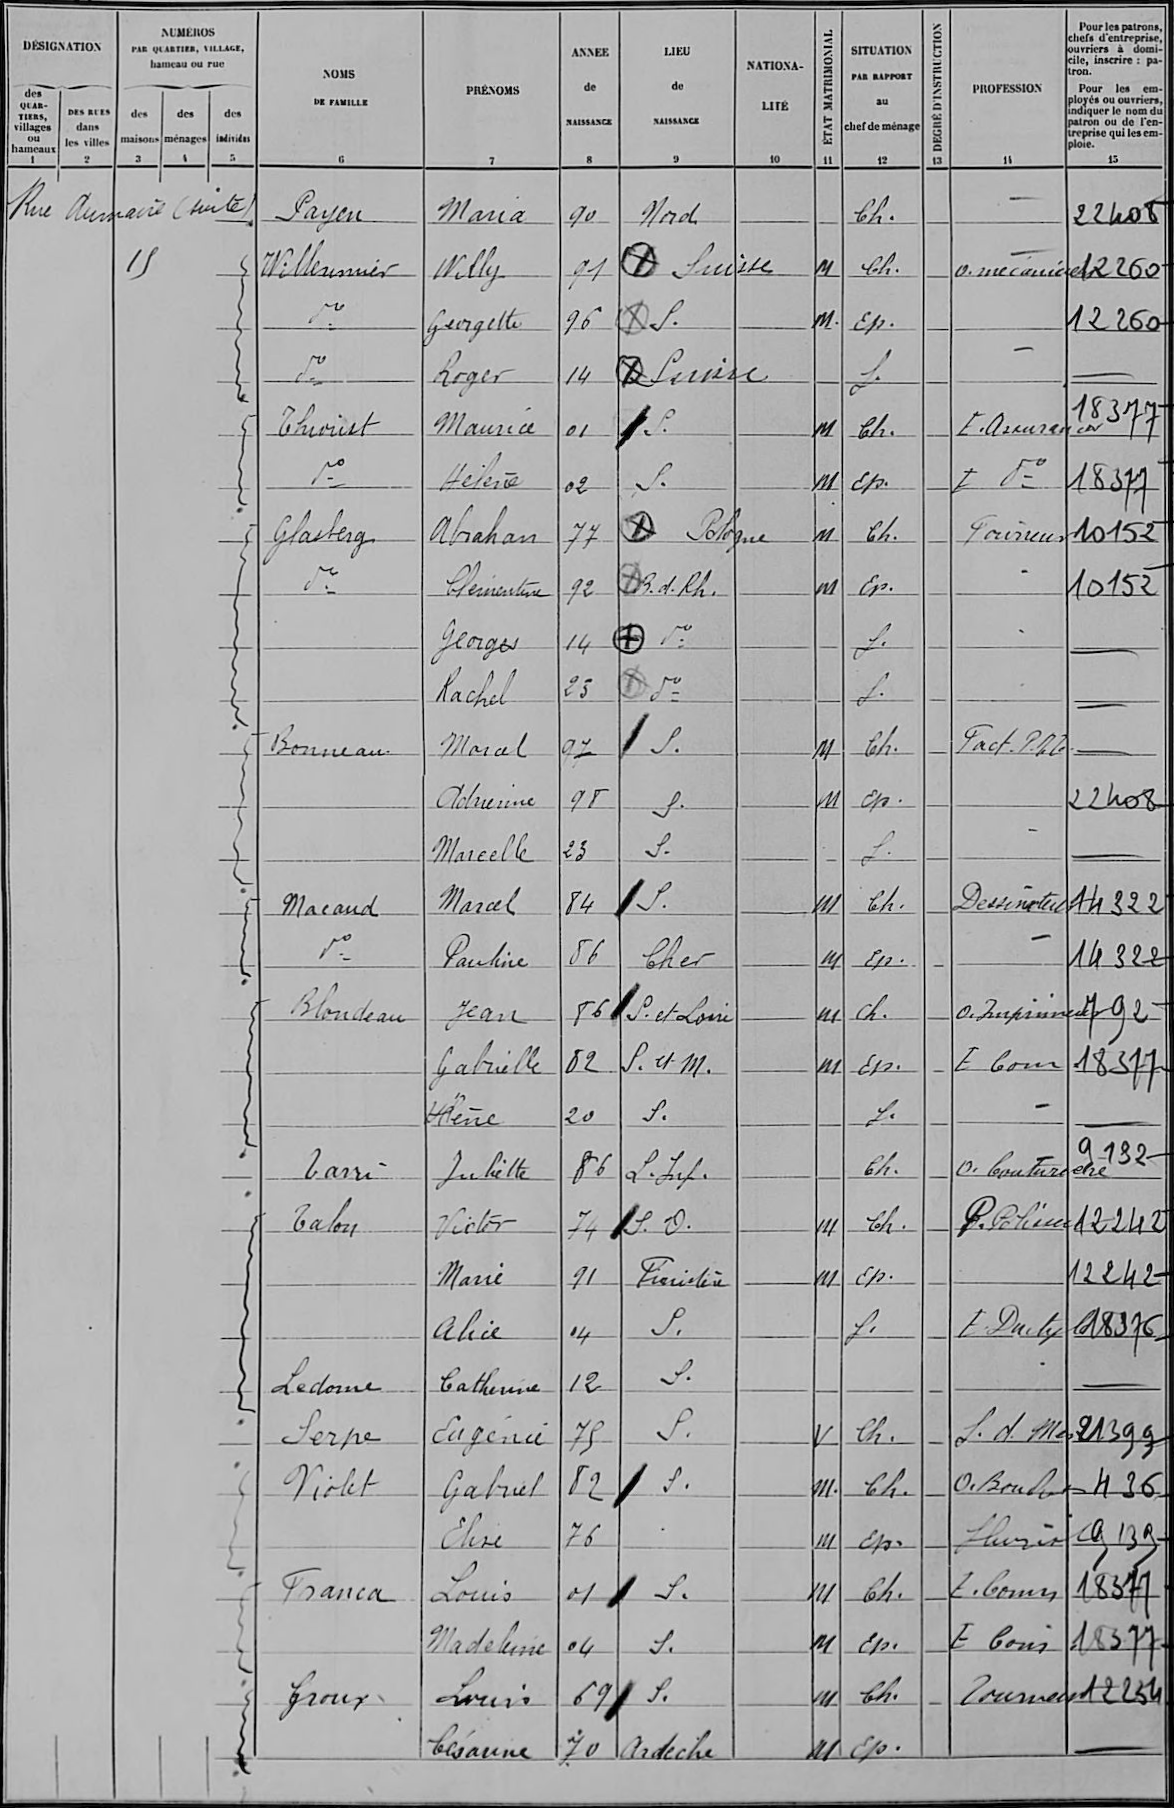Payen/Maria/90/Nord/¤/¤/Ch /¤/¤/22408
Willeumier/Willy/91/Suisse/¤/M/Ch /¤/o mécanicien/12260
d°/Georgette/96/S /¤/M /Ep /¤/¤/12260
d°/Roger/14/Suisse/¤/¤/f /¤/¤/¤
Thivrist/Maurice/01/S /¤/M/Ch /¤/E Assurance/18377
d°/Hélène/02/S /¤/M/Ep /¤/E d°/18377
Glasberg/Abrham/77/Pologne/¤/M/Ch /¤/Fourreur/10152
d°/Clémentine/92/B d Rh /¤/M/ep /¤/¤/10152
¤/Georges/14/d°/¤/¤/f /¤/¤/¤
¤/Rachel/23/d°/¤/¤/S /¤/¤/¤
Bonneau/Marcel/97/S /¤/M/Ch /¤/Fact PTT/¤
¤/Adrienne/98/S /¤/M/ep /¤/¤/22408
¤/Marcelle/23/S /¤/¤/f /¤/¤/¤
Macaud/Marcel/84/S /¤/M/Ch /¤/Dessinateur/14322
d°/Pauline/86/Cher/¤/M/Ep /¤/¤/14322
Blondeau/Jean/86/S et Loire/¤/M/Ch /¤/o Imprimeur/792
¤/Gabrielle/82/S et M /¤/M/Ep /¤/E Com/18377
¤/Hélène/20/S /¤/¤/f /¤/¤/¤
Tarri/Juliette/86/L Inf /¤/¤/Ch /¤/o Couturière/?9132
Talon/Victor/74/S O /¤/M/Ch /¤/P Polisseur/12242
¤/Marie/91/Finistère/¤/M/Ep /¤/¤/12242
¤/Alice/14/S /¤/¤/f /¤/E Dactylo/18376
Ledorne/Catherine/12/S /¤/¤/¤/¤/¤/¤
Serpe/Eugénie/75/S /¤/V/Ch /¤/f d Men/21399
Violet/Gabriel/82/S /¤/M /Ch /¤/O Boucher/436
¤/Elise/76/¤/¤/M/Ep /¤/fleuriste/9139
Franca/Louis/01/S /¤/M/Ch /¤/E Comm/18377
¤/Madeleine/04/S /¤/M/Ep /¤/E Com/18377
Groux/Louis/69/S /¤/M/Ch /¤/tourneur/12254
¤/Césarine/70/Ardèche/¤/M/Ep /¤/¤/¤
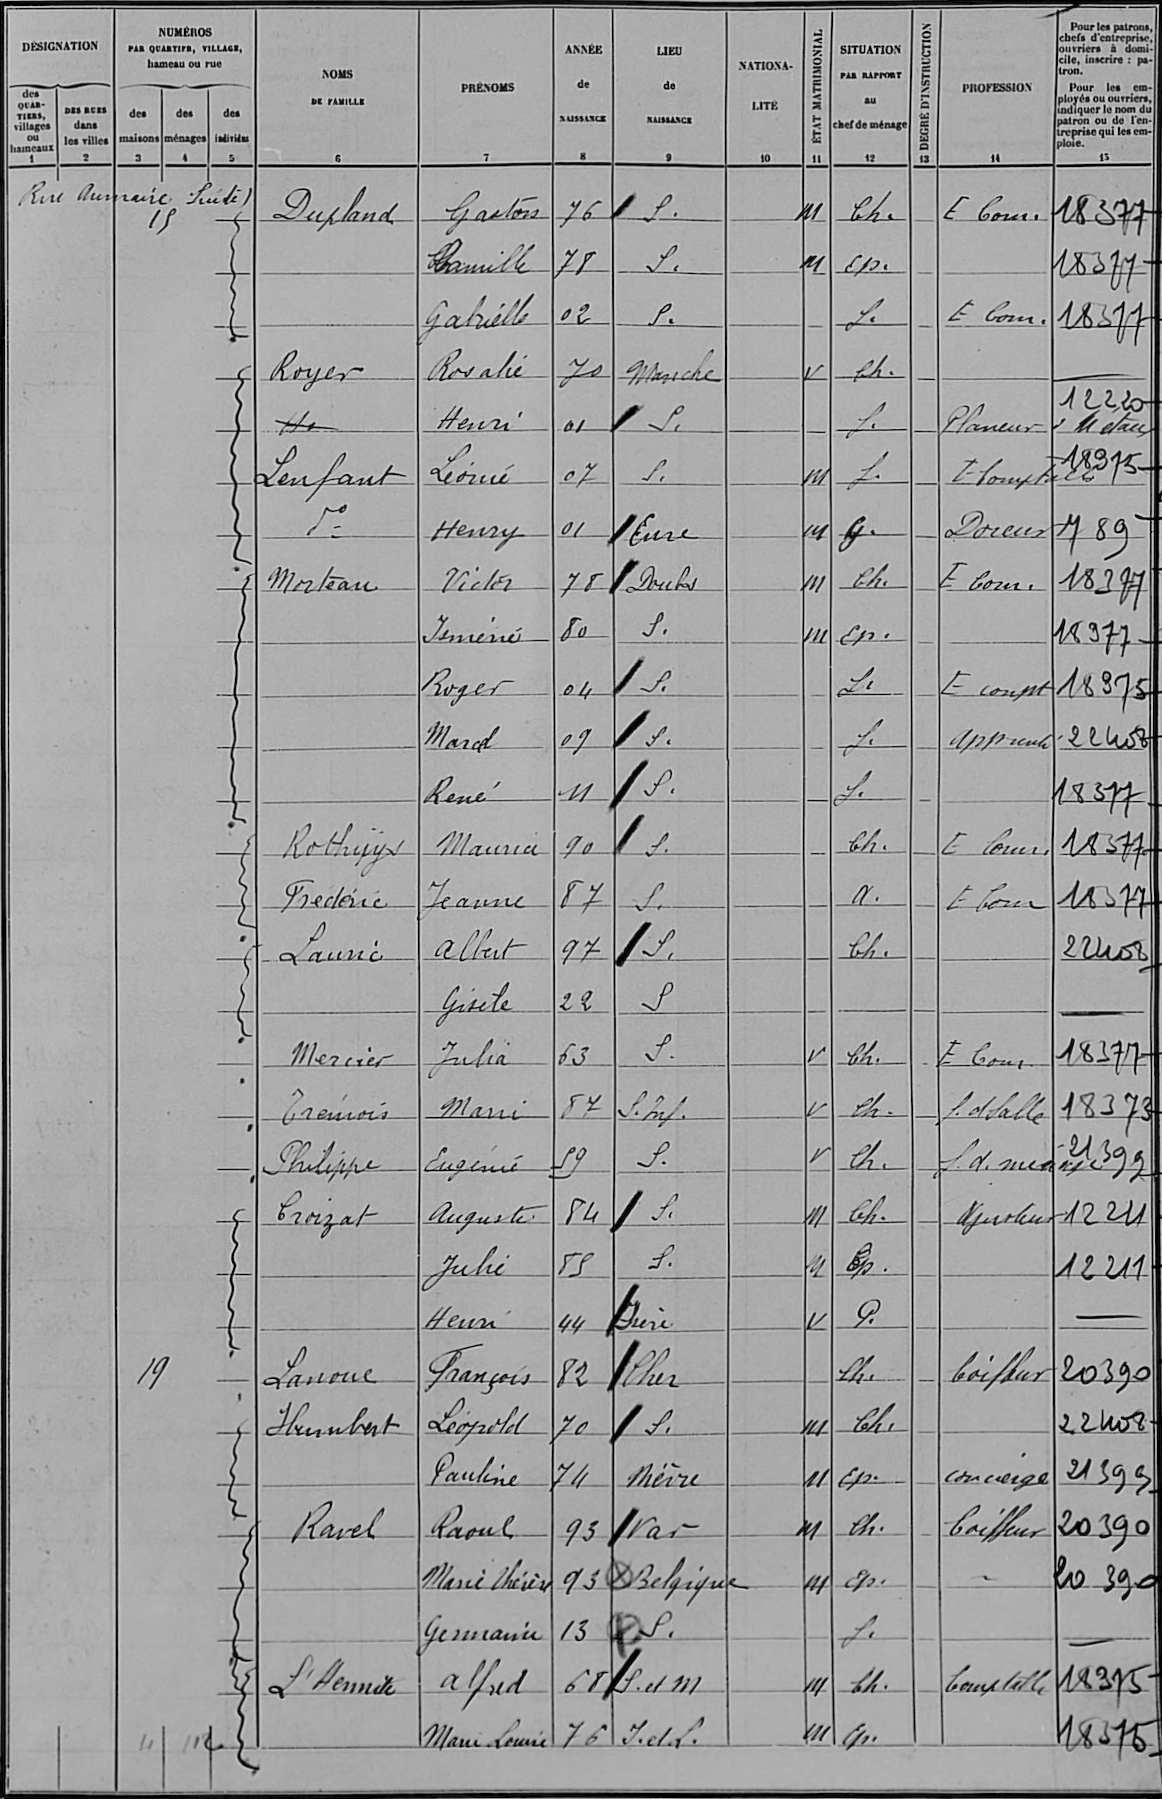Dupland/Gaston/76/S /¤/M/Ch /¤/E Com/18377
¤/Camille/78/S /¤/M/Ep /¤/¤/18377
¤/Gabrielle/02/S /¤/¤/f /¤/E Com /18377
Royer/Rosalie/70/Manche/¤/V /Ch /¤/¤/¤
¤/Henri/01/S /¤/¤/f /¤/Planeur de Métaux/?12220
Lenfant/Léonie/07/S /¤/M/f /¤/E Comptable/?18375
d°/Henry/01/Eure/¤/M/g /¤/Doreur/789
Morteau/Victor/78/Doubs/¤/M/Ch /¤/E Com /18377
¤/Isménie/80/S /¤/M/Ep/¤/¤/18377
¤/Roger/04/S /¤/¤/f /¤/E compt/18375
¤/Marcel/09/S /¤/¤/f /¤/Apprenti/22408
¤/René/11/S /¤/¤/f /¤/¤/18377
Rothijys/Maurice/90/S /¤/¤/Ch /¤/E Com /18377
Frédéric/Jeanne/87/S /¤/¤/a /¤/E Com/18377
Lauric/Albert/97/S /¤/¤/Ch /¤/¤/22408
¤/Gisèle/22/S/¤/¤/¤/¤/¤/¤
Mercier/Julia/63/S /¤/v/Ch /¤/E Com/18377
Trémois/Marie/87/S Inf/¤/v/ch /¤/f d Salle/18373
Philippe/Eugénie/59/S /¤/V/Ch /¤/f d ménage/21399
Croizat/Auguste/84/S /¤/M/Ch /¤/Ajusteur/12211
¤/Julie/85/S /¤/M/Ep /¤/¤/12211
¤/Henri/44/Isère/¤/V/P /¤/¤/¤
Lanoue/François/82/Cher/¤/¤/Ch /¤/Coiffeur/20390
Humbert/Léopoled/70/S /¤/M/Ch /¤/¤/22408
¤/Pauline/74/Nièvre/¤/M/Ep /¤/concierge/21399
Ravel/Raoul/93/Var/¤/M/Ch /¤/Coiffeur/20390
¤/Marie Thérèse/93/Belgique/¤/M/Ep /¤/¤/20390
¤/Germain/13/S /¤/¤/f /¤/¤/¤
L'Hermite/Alfred/68/S et M/¤/14/Ch /¤/Comptable/18375
¤/Marie Louise/76/I et L /¤/M/Ep /¤/¤/18375